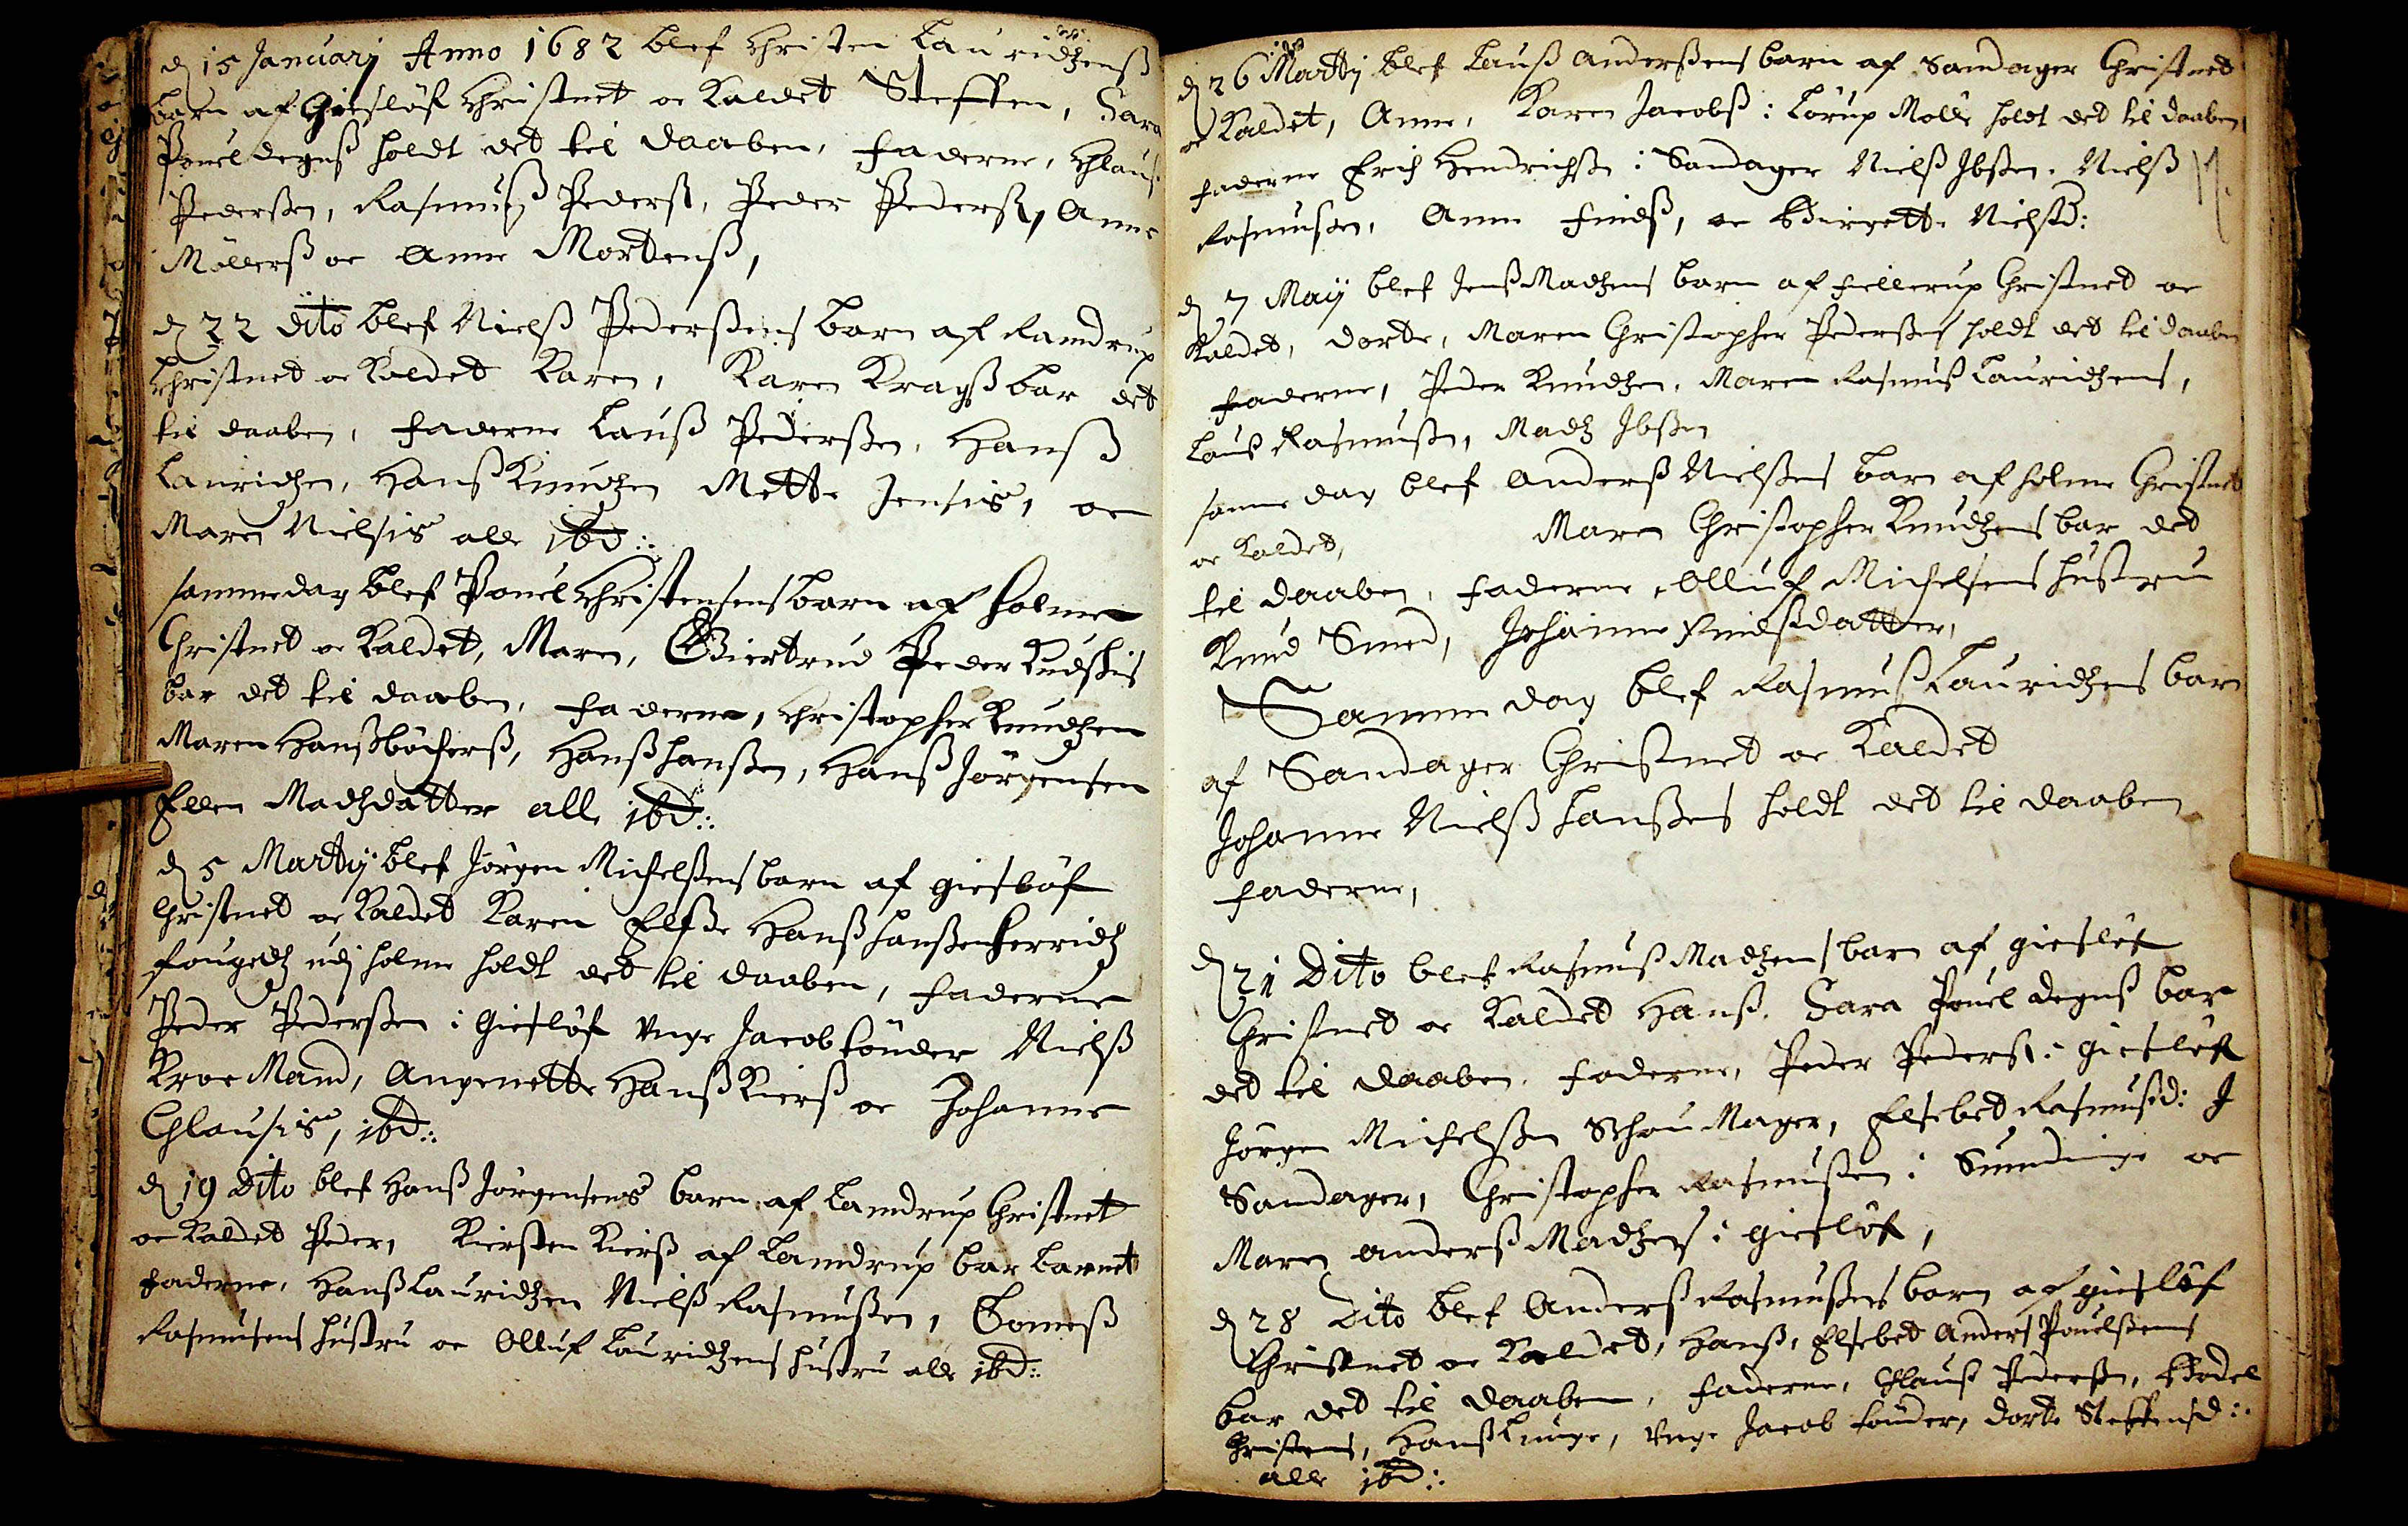d 15 Januarij Anno 1682 blef Christen Lauridtzenss
baren af Giesløf Christnet oc kaldet Steffen, Sara
Pouel Degnss holdt det til Daaben, Faderne, Chlaus
Pederssen, Rasmuss Peders, Peder Peders, Anne
Møllerss oc Anne Mortenss,
d 22 dito bleff Nielss Pederssens barn af Ramdrup
Christnet oc kaldet Karen, Karen Kragss bar det
til daaben, Faderne Lauss Pederssen, Hanss
Lauridtzen, Hanss Knudtzen Mette Jensis, oc
Maren Nielsis alle ibd:.
sammedag blef Pouel Christensens barn af Holme
Christnet oc kaldet, Maren, Giertrud Peder Kudskis
bar det til daaben, Fadernne, Christopher Knudtzen
Maren Hanss Bøcherss, Hanss Hanssen, Hanss Jørgensen
Peder Madtzdatter all ibd:.
d 5 Martij blef Jørgen Michelssens barn af Giesløf
christnet oc kaldet Karen Elsse Hanss Hanssen Herridtz
fougedtz udi holme holdt det til Daaben, Faderne
Peder Pederssen i Giesløf vnge Jacob tønder Nielss
Kroe Mand, Angenette Hanss Kierss oc Johanne
Chlausis, ibd:.
d 19 Dito blef Hanss Jørgensens barn af Lamdrup Christent
oc kaldet Peder Kiersten Kierss af Lamdrup bar barnet
Faderer, Hanss Lauridtzen Niels Rasmussen, Tomess
Rasmusens Hustru oc Olluf Lauridtzens hustru alle ibd:.
17.
d 26 Martij blef Lauss Anderssens barn af Sandager Christnet
oc kaldet, Anne, Karen Jacobs i Lørup Mølle holdt det til daaben
Faderen Erich Hendrichs i Sandager Nielss Ibssen. Nielss
Rasmusen, Anne Finds, oc Birrette Nielsd:
d 7 Maij blef Jenss Madtzens barn af Fiellerup Christned oc
kaldet, Dorte, Maren Christopher Pederssens holdt det til daaben
Faderne, Peder Knudtzen, Maren Rasmuss Lauridtzens,
Lauss Rasmusen, Madtz Ibssen
same dag blef Anderss Nielssens barn af holme Christenet
oc kaldet
Maren Christopher Knudtzens bar det
til daaben, Faderne Olluf Michelsens hustru
Knud Smed; Johanne Findssdaatter,
Samme dag blef Rasmus Lauridtzes barn
af Sandager Christnet oc kaldet
Johanne Nielss Hansens holdt det til daaben
Faderne,
d 21 Dito blef Rasmuss Madtzens barn af giesløf
Christnet oc kaldet Hanss, Sara Pouel Degnss bar
det til daaben, Faderne, Peder Peders i Giesløf
Jørgen Michelssen Schou Mager, Elsebet Rasmussd: I
Sandager, Christopher Rasmussen i Suendinge oc
Maren Anderss Madtzens i Giesløf,
d 28 Dito blef Anderss Rasmussens barn af giesløf
Christnet oc koeldet, Hanss, Elsebet Anders Pouelssens
bar det til daaben, Faderne, Chlauss Pederssen, Bodel
Christens, Hanss L##ge, vnge Jacob tønder, Dorte Steffensd:
alle ibd:.
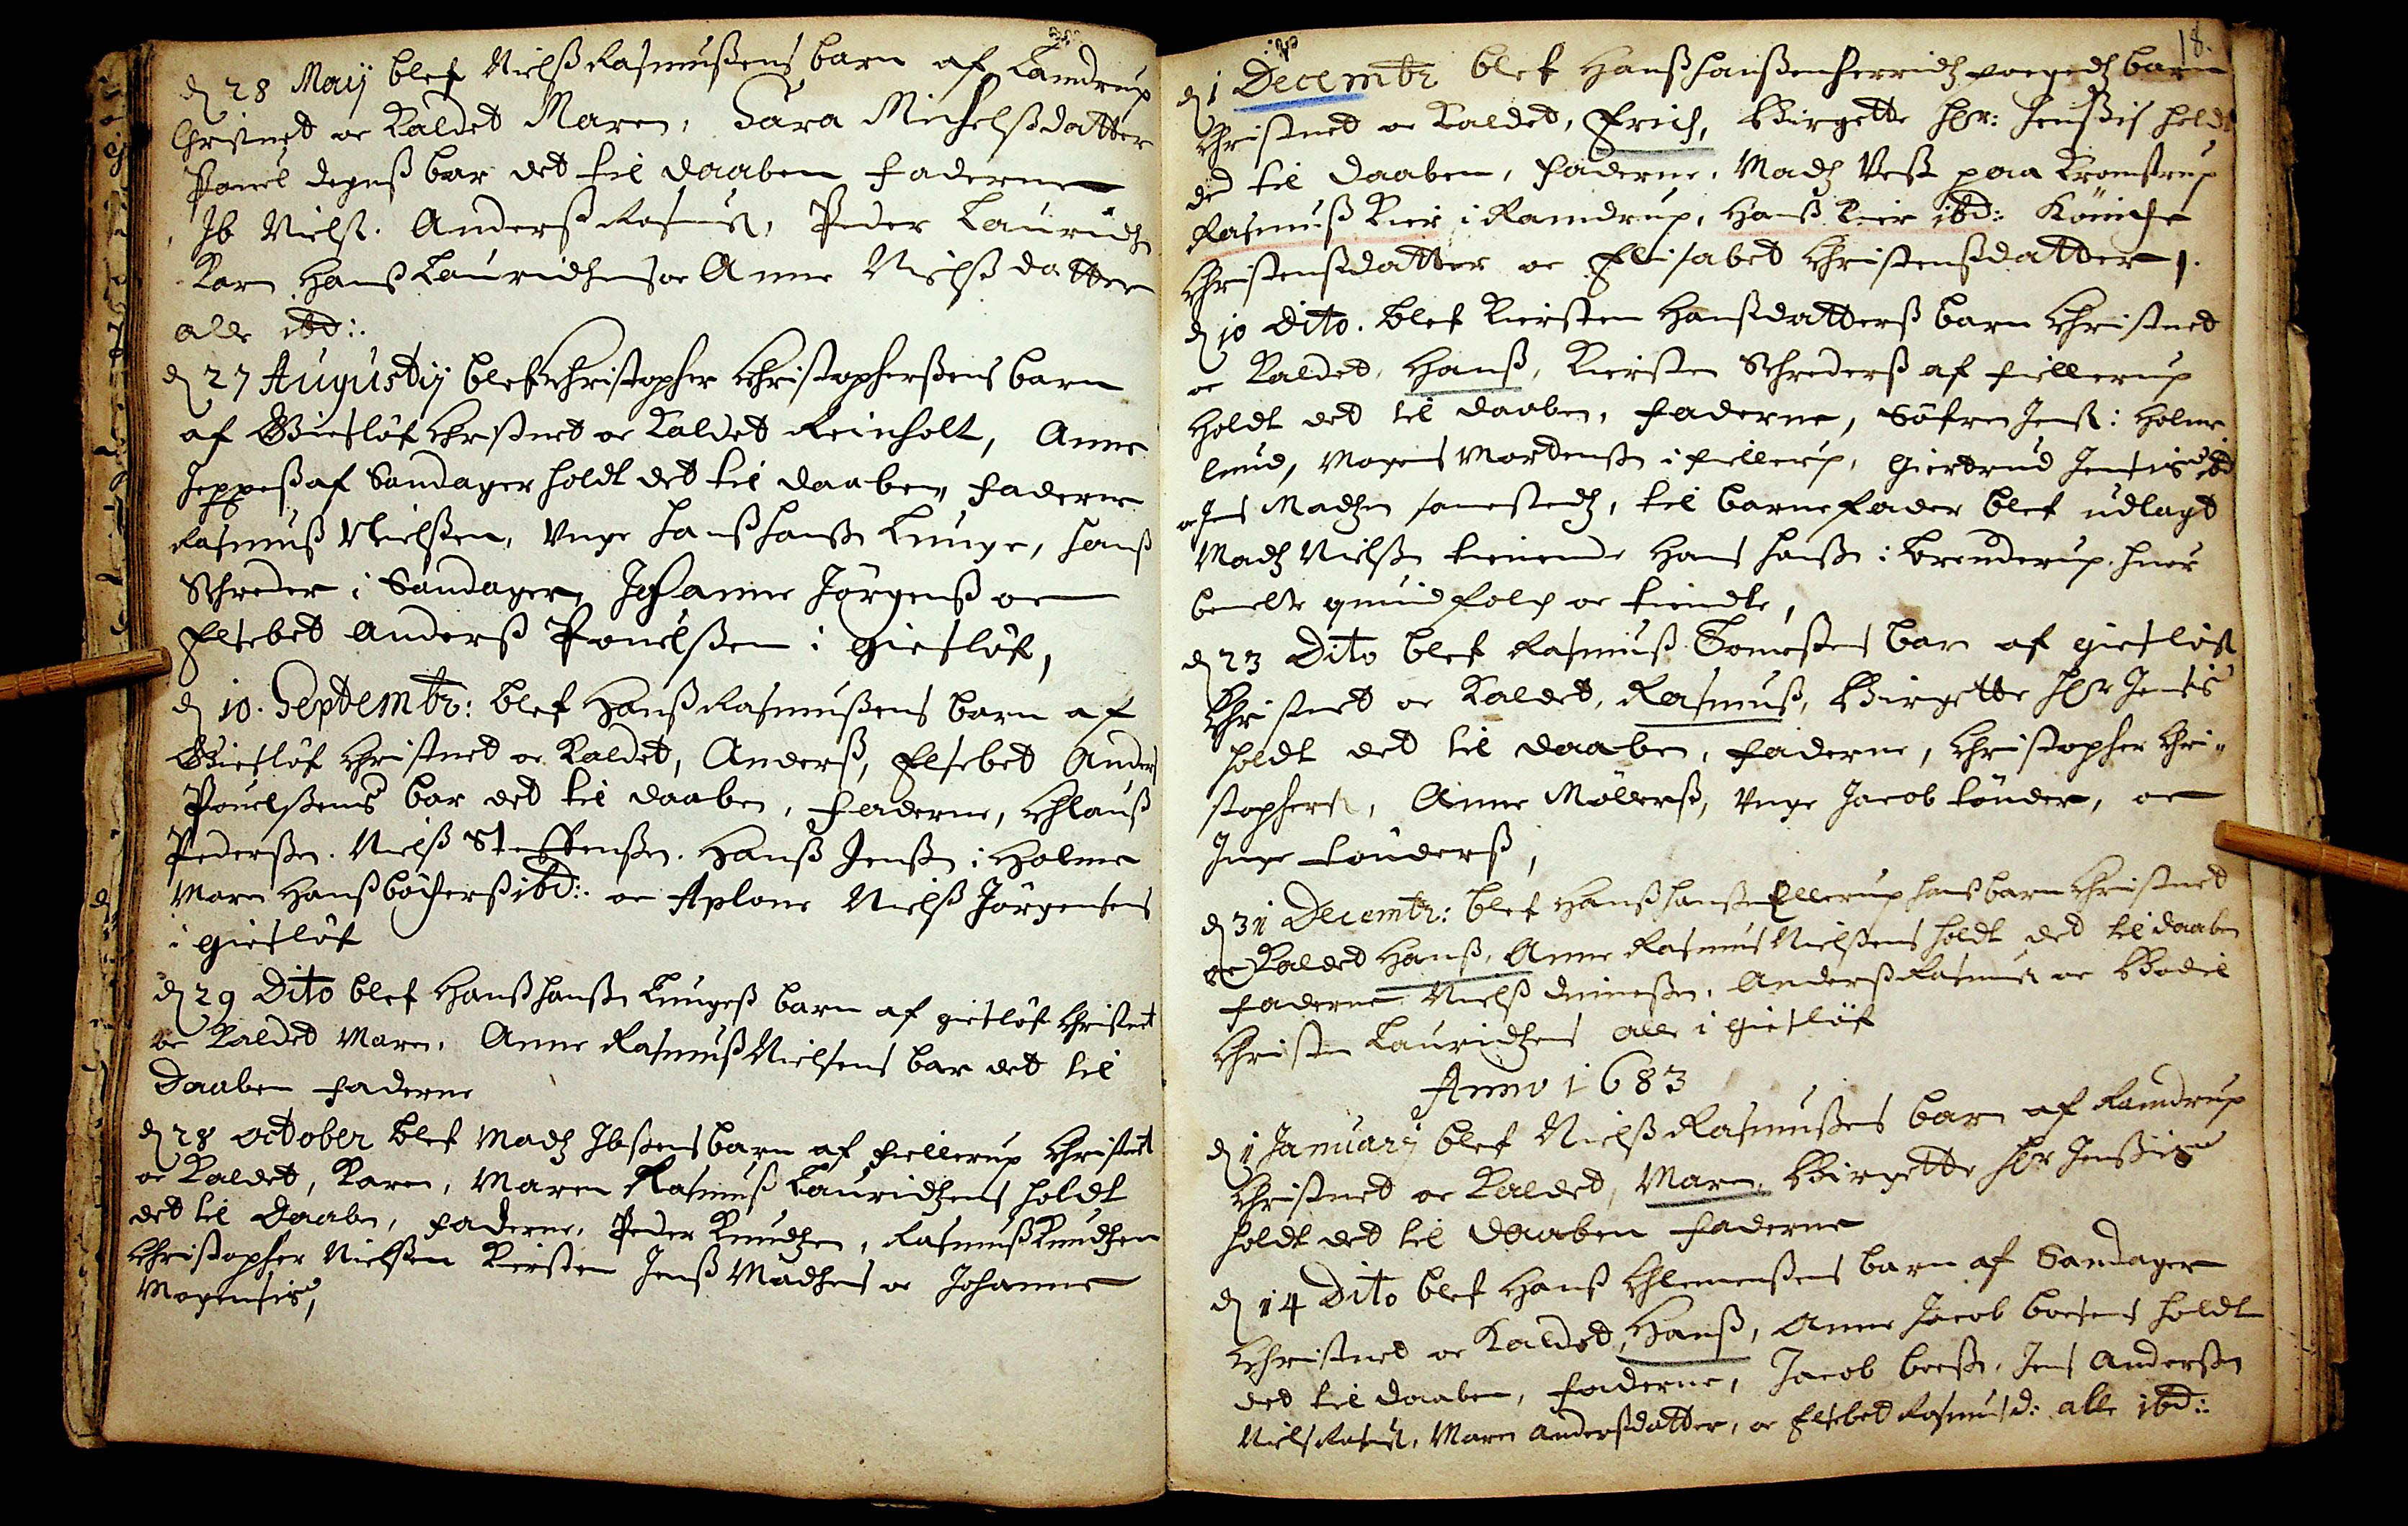d 28 Maij blef Nielss Rasmussens barn af Lamdrup
Christnet oc kaldet Maren, Sara Michelssdatter
Poueel Degns bar det til daaben. Faderne
Ib Niels. Anderss Rasmus Peder Lauridtz
kone Hans Lauridtzens oc Anne Nielsdatter
alle ibd:.
27 Augustij blef Christopher Christopherssens barn
af Giesløf Christnet oc kaldet Reinholt, Anne
Jeppenss af Sandager holdt det til daaben, Faderne
Rasmuss Nielssen, vnge Hanss Hanss Lunge, Hanss
Schreder i Sandager, Johaanne Jørgenss oc
Elsebet Anderss Pouelssen i Giesløf,
d 10. Septembr: blef Hanss Rasmussens barn af
Giesløf Christnet oc kaldet Anderss, Elsebet Anders
Pouelssens bar det til daaben, Faderne, Chlauss
Pederssen. Niels Steffenssen. Hanss Jenssen i Holme
Maren Hanss bøcherss ibd:. oc Aplone Nielss Jørgensens
i Giesløf
d 29 Dito blef Hanss Hanssen Lungess barn af gietløf Christent
oc kaldet Maren. Anne Rasmuss Nielsens bar det til
Daaben Faderne
d 28 october blef Madtz Ibsens barn af Fiellerup Christens
oc kaldet, Karen, Maren Rasmuss Lauridtzens holdt
det til Daaben, Faderne, Peder Knudtzen, Rasmuss Knudtzen
Christopher Nielssen Kiersten Jenss Madtzens oc Johanne
Mogensis,
18.
d 1 Decembr blef Hanss Hanssen Herridtzfoegedtz barn
Christned oc kaldet, Erich, Birgette hlr: Jenssis holdt
det til Daaben, Faderne, Madtz Vess paa
Rasmuss Kier i Ramdrup, Hanss Kier ibd:. Køniche
Christensdatter oc Elisabet Christensdatter,
d10 Dito. blef Kiersten Hanssdatterss barn Christnet
oc kaldet, Hanss, Kiersten Schreders af Fiellerup
Holdt det til daaben, Faderne, Søfren Jenss i Holme
lund, Mogens Mortenssen i Fiellerp, Giertrud Jenfis ibd
oc Jens Madtzen samestedts, til barnefader blef udlagt
Madtz Nielssen tienende Hans Hanssen i brenderup, huer
beneldte quindfolk oc tiendte
d 23 Dito blef Rasmuss Tomessens barn af giesløf
Christnet oc kaldet, Rasmuss, Birgette hlr Jensis
holdt det til daaben, Faderne, Christopher Chri¬
stophers, Anne Møllerss, vnge Jacob tønder, oc
Inger tønderss
d 31 Decembr: blef Hanss Hanssen fiellerup hans barn Christnet
oc kaldet Hanss, Anne Rasmus Nielssens holdt det til daaben
Faderne Niels Dinnessen, Anders Rasmus oc Bodil
Christen Lauridtzens alle i giesløf
Anno 1683
d 1 Januarij blef Nielss Rasmussens barn af Ramdrup
Christned oc Kaldet, Maren, Birrette hlr Jenssis
holdt det til Daaben Faderne
d 14 Dito blef Hanss Chlemenssens barn af Sandager
Christned oc kaldet, Hanss, Anne Haeob Boesens holdt
det til daaben, Faderne, Jacob boess, Jens Anderssen
Niels Rasmus, Maren Andersdatter, oc Elsebet Rasmusd: alle ibd:.